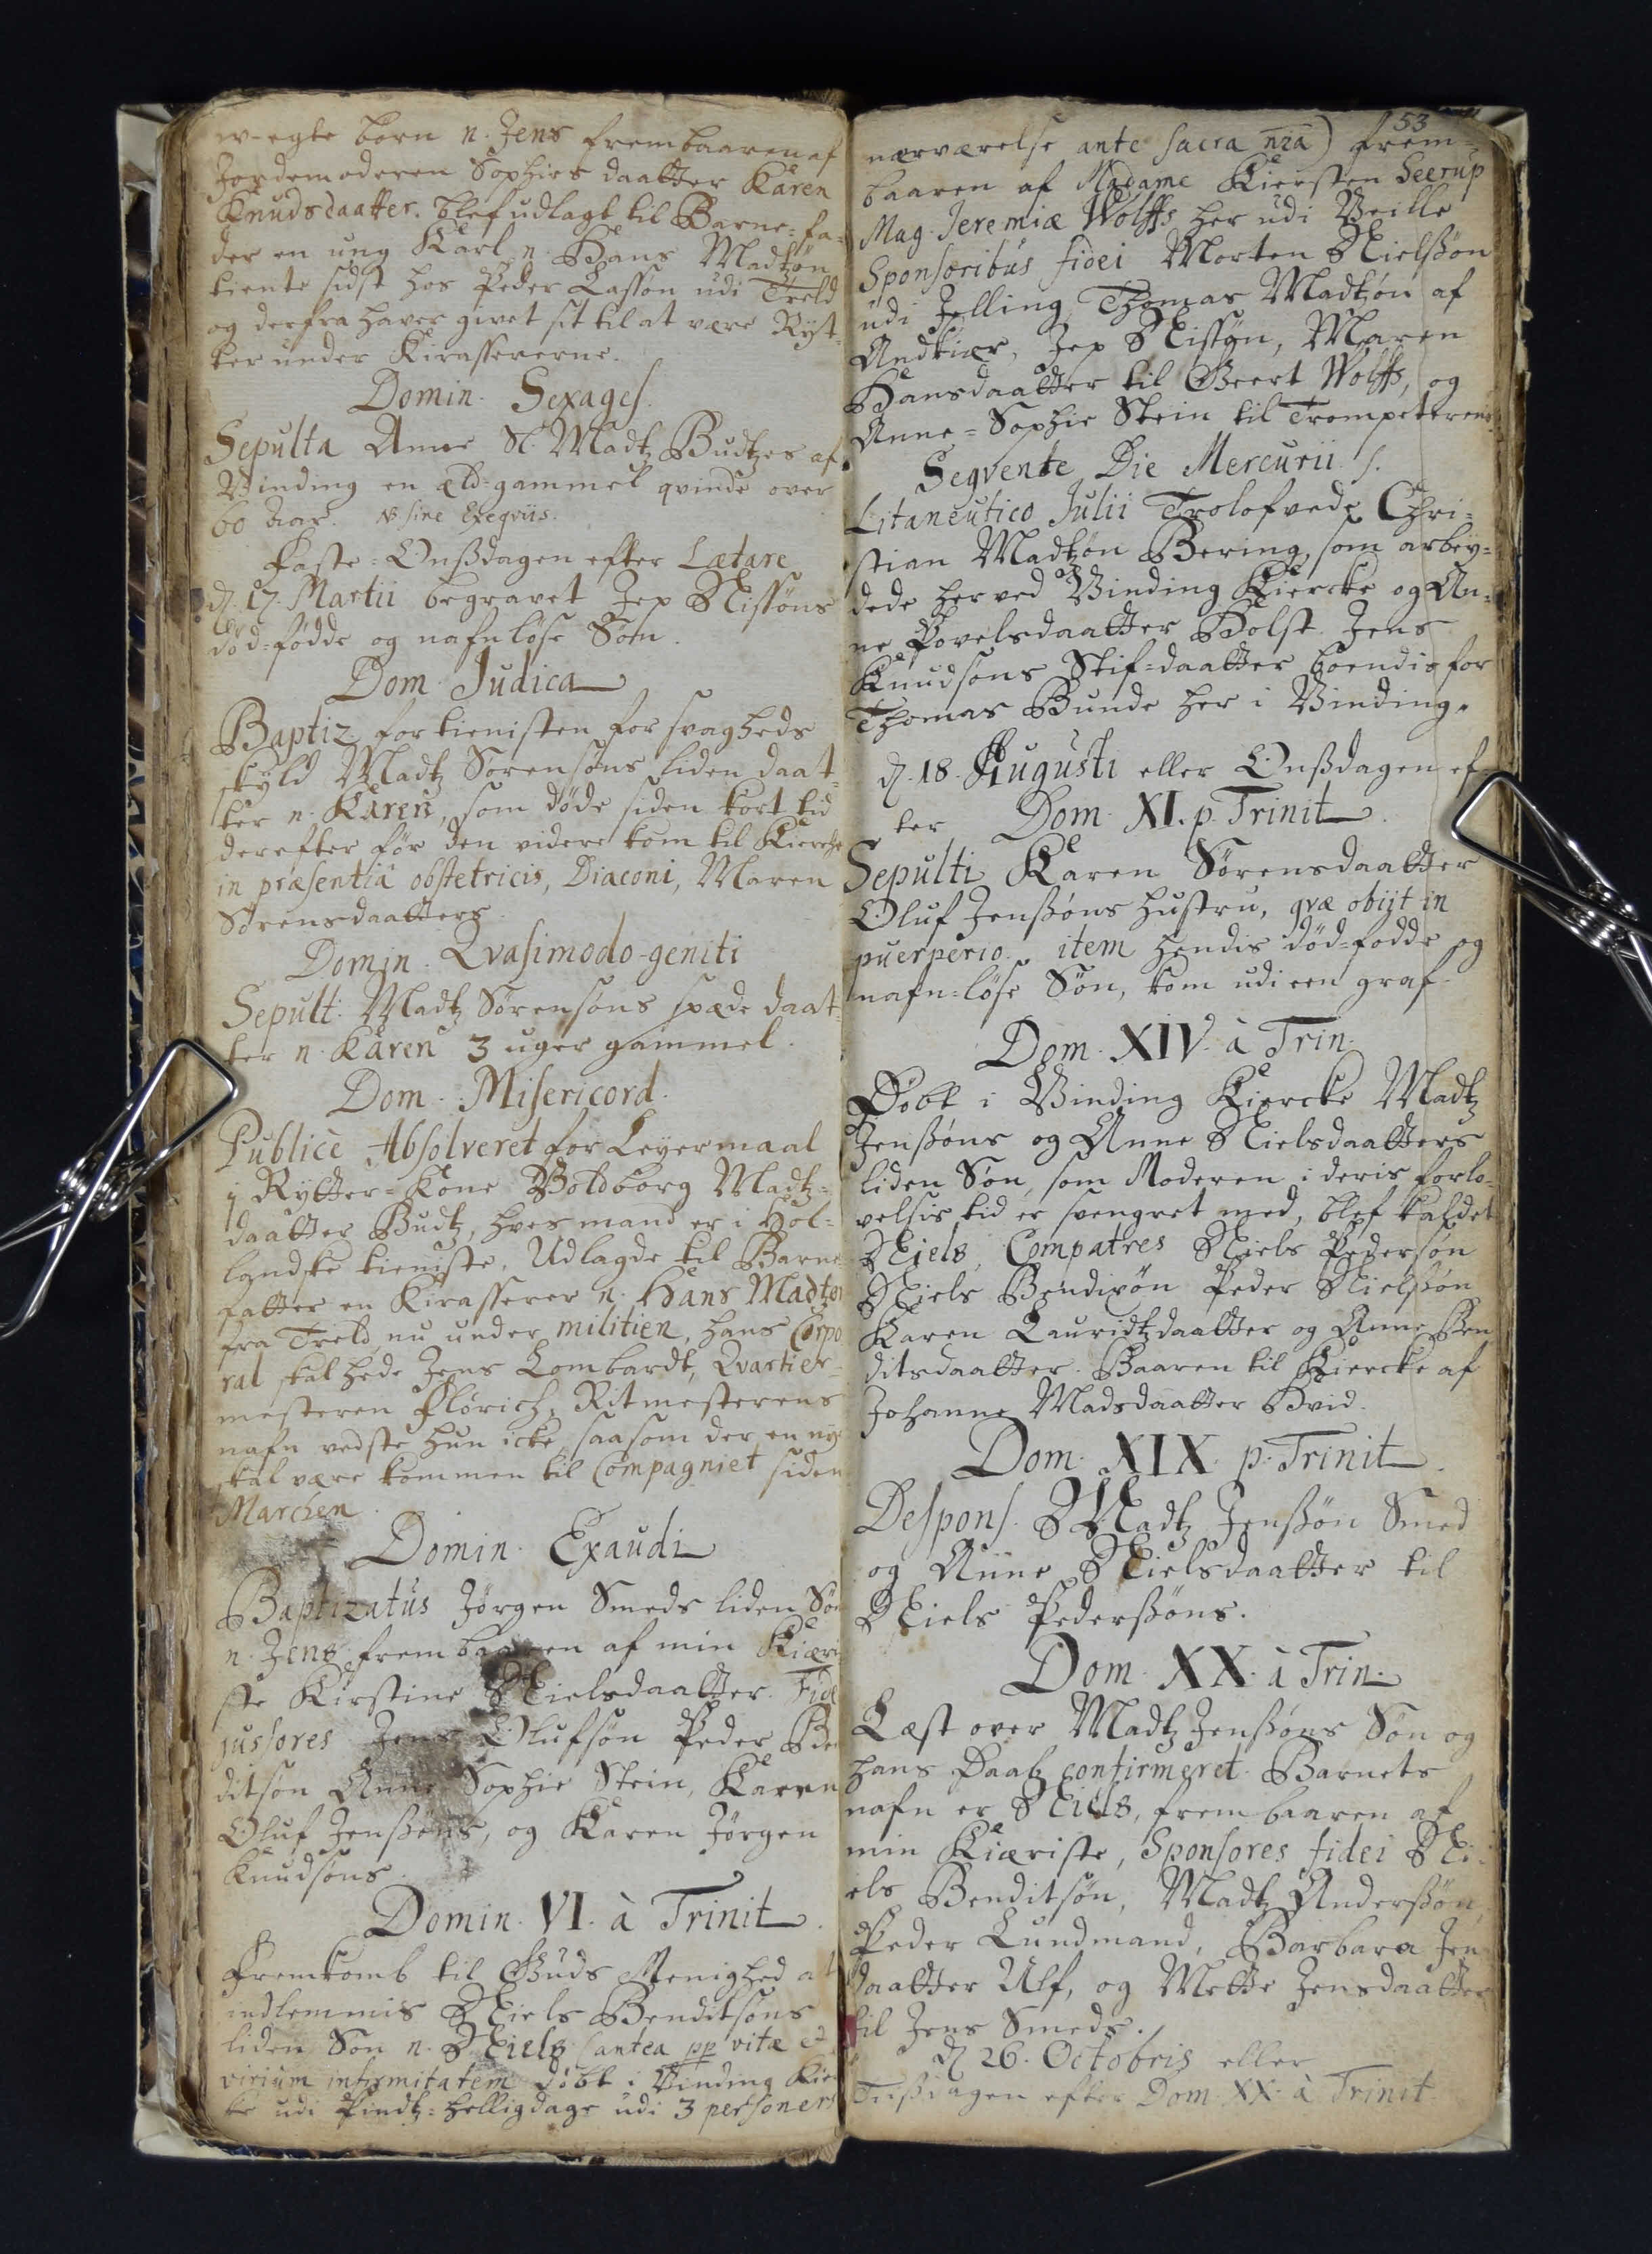w=egte barn n. Jens frembaaren af
Jordemoderen Sophies daatter Karen 
Knudsdaatter. blef udlagt til Barne=fa¬
der en ung Karl n. Hans Madtzøn
tiente sidst hos Peder Lassøn udi Treld 
og derfra haver givet sit til at være Ryt¬
ter under Kirassererne.
Domin Sexages.
Sepulta Anne Sl. Madtz Budtzes af
Vinding, en æld=gammel qvinde over
60 Aar. NB. sine Exeqviis.
Faste = Onsdagen efter Lætare
d. 17 Martii. begravet Jep Nissøns 
død=fødde og nafnløse Søn
Dom. Judica
Baptiz for tienisten for svagheds
skyld Madtz Sørensøns liden daat¬
ter n. Karen, som døde siden kort tid
derefter før den videre kom til Kirche 
in præsentia obstetricis, Diaconi, Maren
Sørensdaatters
Domin. Qvasimodo-geniti
Sepult. Madtz Sørensøns spæde daat¬
ter n. Karen 3 uger gammel
Dom. Micericord
Publice Absolveret for Leyermaal
1 Rytter = Kone Voldborg Madtz¬
daatter Budtz, hvis mand er i Hol¬
landske tieniste, Udlagde til Barne¬
fader en Kirasserer n. Hans Madtzøn
fra Treld, nu under militien, hans Corpo
ral skal hede Jens Lombardt, Qvartier¬
mesteren Flørich, Ritmesterens
nafn vedste hun icke, saa som der en ny 
skal være kommen til Compagniet siden
Marchen.
Domin. Exaudi.
Baptizatus Jørgen Smeds liden Søn
n. Jens, frembaaren af min Kiæri
ste Kirstine Nielsdaatter.
jussores Jens Olufsen Peder Ben
ditsøn Anne Sophie Stein, Karen
Oluf Jenssøns, og Karen Jørgen
Knudsøns
Domin. VI. a Trinit
Fremkomb til Guds Menighed at
indlemmis Niels Benditsøns
liden Søn n. Niels (antes pp vitæ et
virium infirmitatem døbt i Vinding Kir 
ke udi Pindtz = Helliggade udi 3 personers
53
nærværelse ante sacra nia##) frem¬
baaren af Madame Kiersten Seerup
Mag. Jeremiæ Wolffs her udi Veille
Sponsoribus fidei Morten Nielssøn
udi Jelling, Thomas Madtzøn af
Andkiær, Jep Nissøn, Maren
Hansdaatter til Geert Wolffs, og
Anne = Sophie Stein til Trompeterens
Seqvente Die Mercurii. s
Litaneutico Julii. Trolofvede Chri¬
stian Madtzøn Bering, som arbey¬
dede her ved Vinding Kiercke og An¬
ne Povelsdaatter Holst. Jens
Knudsøns Stif=daatter boendis for
Thomas Bunde her i Vinding.
d. 18 Augusti eller Onssdagen ef¬
ter
Dom. XI. p. Trinit.
Sepulti Karen Sørensdaatter
Oluf Jenssøns hustru, qvæ obiit in
puerperio item hendis død=fødde og 
nafn=løse Søn, kom udi een graf.
Dom. XIV a Trin
Døbt i Vinding Kiercke Madtz
Jenssøns og Anne Nielsdaatters
liden Søn, som Moderen i deris forlo¬
velsis tid er svangret med, blef kaldet
Niels, Compatres Niels Pedersøn
Niels Bendixøn Peder Nielssøn
Karen Lauridtzdaatter, og Anne Ben
ditsdaatter. Baaren til Kiercke af
Johanne Madsdaatter Hvid
Dom. XIX p. Trinit
Despons. Madtz Jenssøn Smed
og Anne Nielsdaatter til
Niels Pederssøns.
Dom. XX. a Trin.
Læst over Madtz Jenssøns Søn og
hans Daab confirmeret. Barnets
nafn er Niels, frembaaren af
min Kiæriste, Sponsores fidei Ni¬
els Benditsøn. Madtz Anderssøn,
Peder Lundmand, Barbara Jen
daatter Ulf, og Mette Jensdaatter
til Jens Smeds
d 26. Octobris eller
Tiissdagen efter Dom. XX. a Trinit.
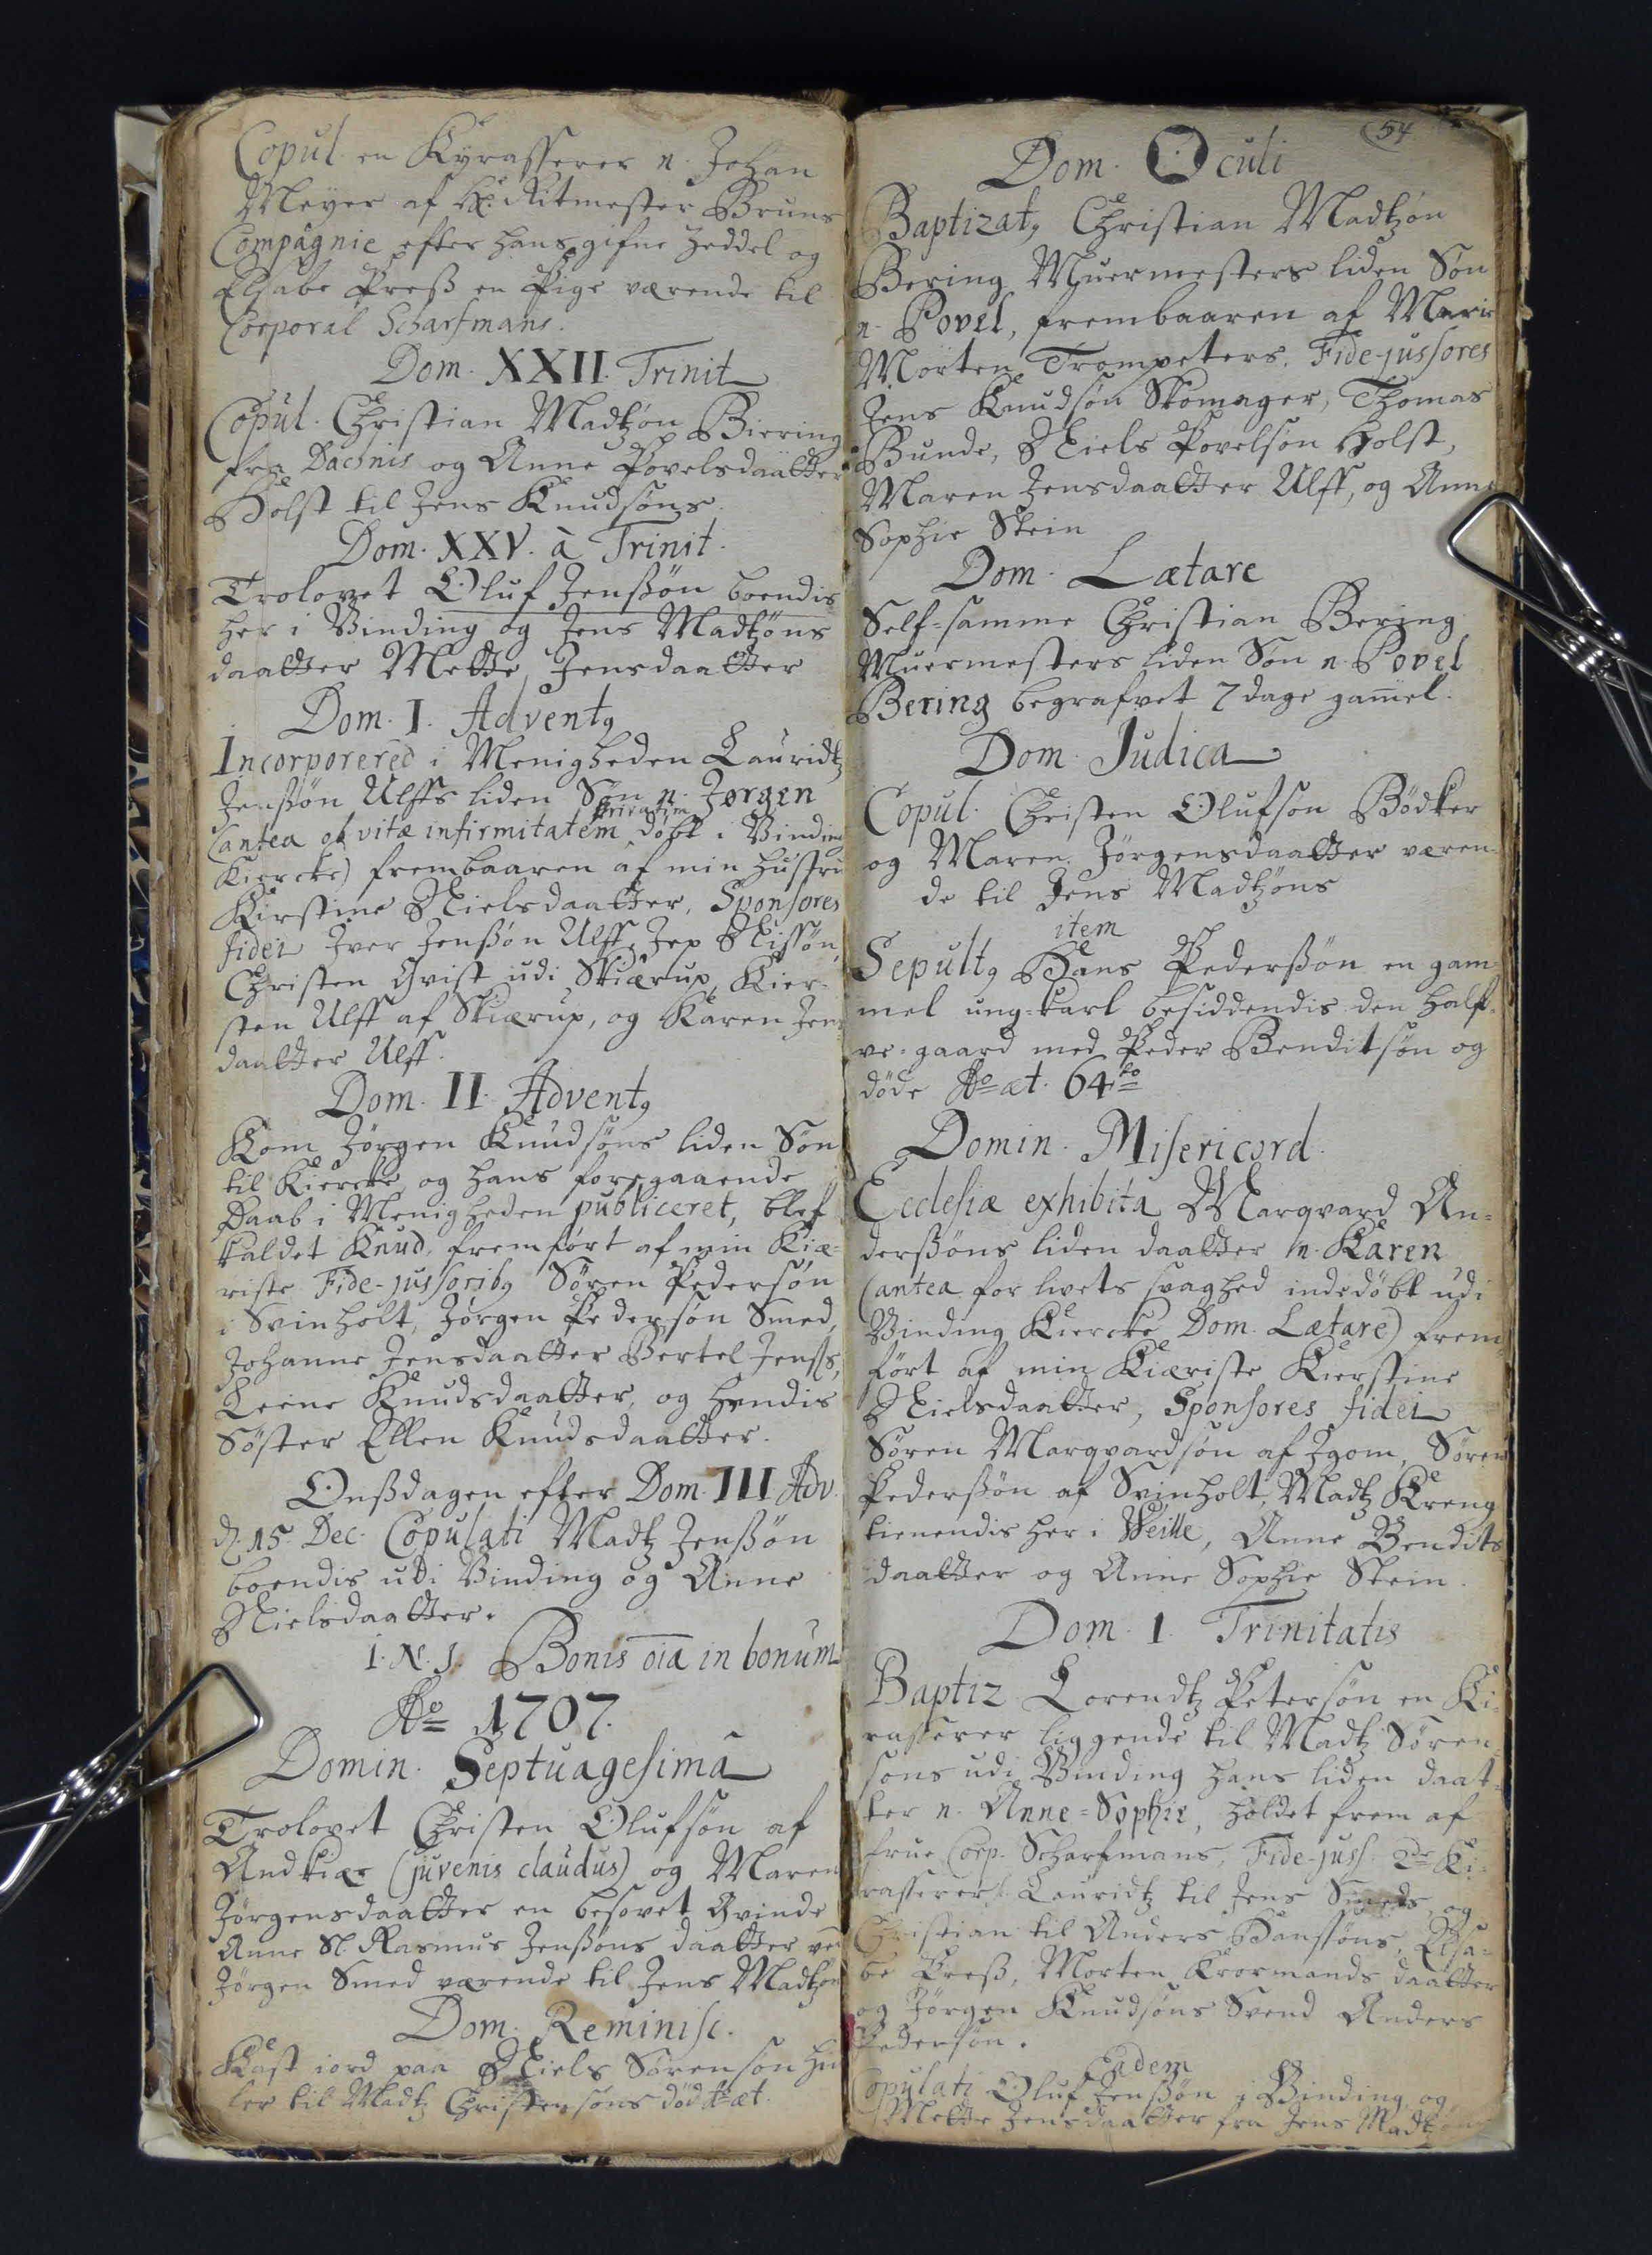Copul. en Kyrasserer n. Johan
Meyer af Hr. Ritmester Bruns 
Compagnie efter hans gifne Zeddel og
Elsabe Press en Pige værende til
Corporal Scharfmans.
Dom. XXII. Trinit
Copul. Christian Madtzøn Biering
fra Dachnes og Anna Povelsdaatter 
Holst til Jens Knudsøns
Dom. XXV. a Trinit
Trolovet Oluf Jenssøn boendis
her i Vinding og Jens Madtzøns
daatter Mette Jensdaatter.
Dom. I. Adventq
Incorporered i Menigheden Lauridtz
Jenssøn Ulffs liden Søn n. Jørgen
##eieatim
(antea ob vitæ infirmitatem døbt i Vinding
Kiercke) frembaaren af min hustru
Kirstine Nielsdaatter. Sponsores
fidei Iver Jenssøn Ulff, Jep Nissøn,
Christen Qvist udi Skiærup, Kier¬
sten Ulff af Skiærup, og Karen Jens¬
daatter Ulff.
Dom. II. Adventq
Kom Jørgen Knudsøns liden Søn
til Kiercke og hans foregaaende
Daab i Menigheden publiceret, blef
kaldet Knud, frembaaren af min Kiæ¬
riste Fide-jussoribq Søren Pedersøn
i Svinholt, Jørgen Pedersøn Smed,
Johanne Jensdaatter Bertel Jenss,
Leene Knudsdaatter, og hendis
Søster Ellen Knudsdaatter.
Onssdagen efter Dom. III Adv.
d. 15. Dec. Copulati Madtz Jenssøn
boendis udi Vinding og Anne
Nielsdaatter
I. N. J. Bonis oia in bonum
Ao 1707
Domin Septuagesima
Trolovet Christen Olufsøn af
Andkiær (juvenis claudus) og Maren
Jørgensdaatter, en besovet Qvinde
Anna Sl. Rasmus Jenssøns daatter ved
Jørgen Smed værende til Jens Madtzøn
Dom. Reminisc.
Kast iord paa Niels Sørensøn Hiu
ler til Madtz Christensøns, død Ao æt
54
Dom. Oculi.
Baptizatq Christen Madtzøn
Bering Muermesters liden Søn
n. Povel, frembaaren af Marie
Morten Trompeters. Fide-jussores
Jens Knudsøn Skomager, Thomas
Bunde, Niels Povelsøn Holst,
Maren Jensdaatter Ulff, og Anne
Sophie Stein
Dom. Lætare
Self=samme Christen Bering
Muermesters liden Søn n. Povel
Bering begrafvet 7 dage gammel.
Dom. Judica
Copul. Christen Olufsøn Bødker
og Maren Jørgensdaatter væren¬
de til Jens Madtzøns
item
Sepultq Hans Pederssøn en gam¬
mel ung=karl besiddendis den half¬
ve = gaard med Peder Benditsøn og
døde Ao æt. 64to
Domin Misericord
Ecclesiæ exhibita Marqvard An¬
derssøns liden daatter n. Karen
(antea for livets svaghed indedøbt udi
Vinding Kiercke Dom. Lætare) frem¬
ført af min Kiæriste Kierstine
Nielsdaatter, Sponsores fidei
Søren Marqvardsøn af Igom, Søren
Pederssøn af Svinholt, Madtz Kreng
tienendis her i Weille, Anne Bendits¬
daatter og Anne Sophie Stein
Dom. I Trinitatis
Baptiz. Lorendtz Petersøn en Ki¬
rasserer liggende til Madtz Søren¬
søns udi Vinding hans liden daat¬
ter n. Anne=Sophie, holdet frem af 
frue Corp. Scharfmans, Fide-juss 2de Ki¬
rasserer Lauridtz til Jens Smeds, og
Christian til Anders Hanssøns, Elsa¬
be Press, Morten Krormands daatter
og Jørgen Knudsøns Svend Anders 
Pedersøn.
Eadem
Copulati. Oluf Jenssøn i Vinding og
Mette Jensdaatter fra Jens